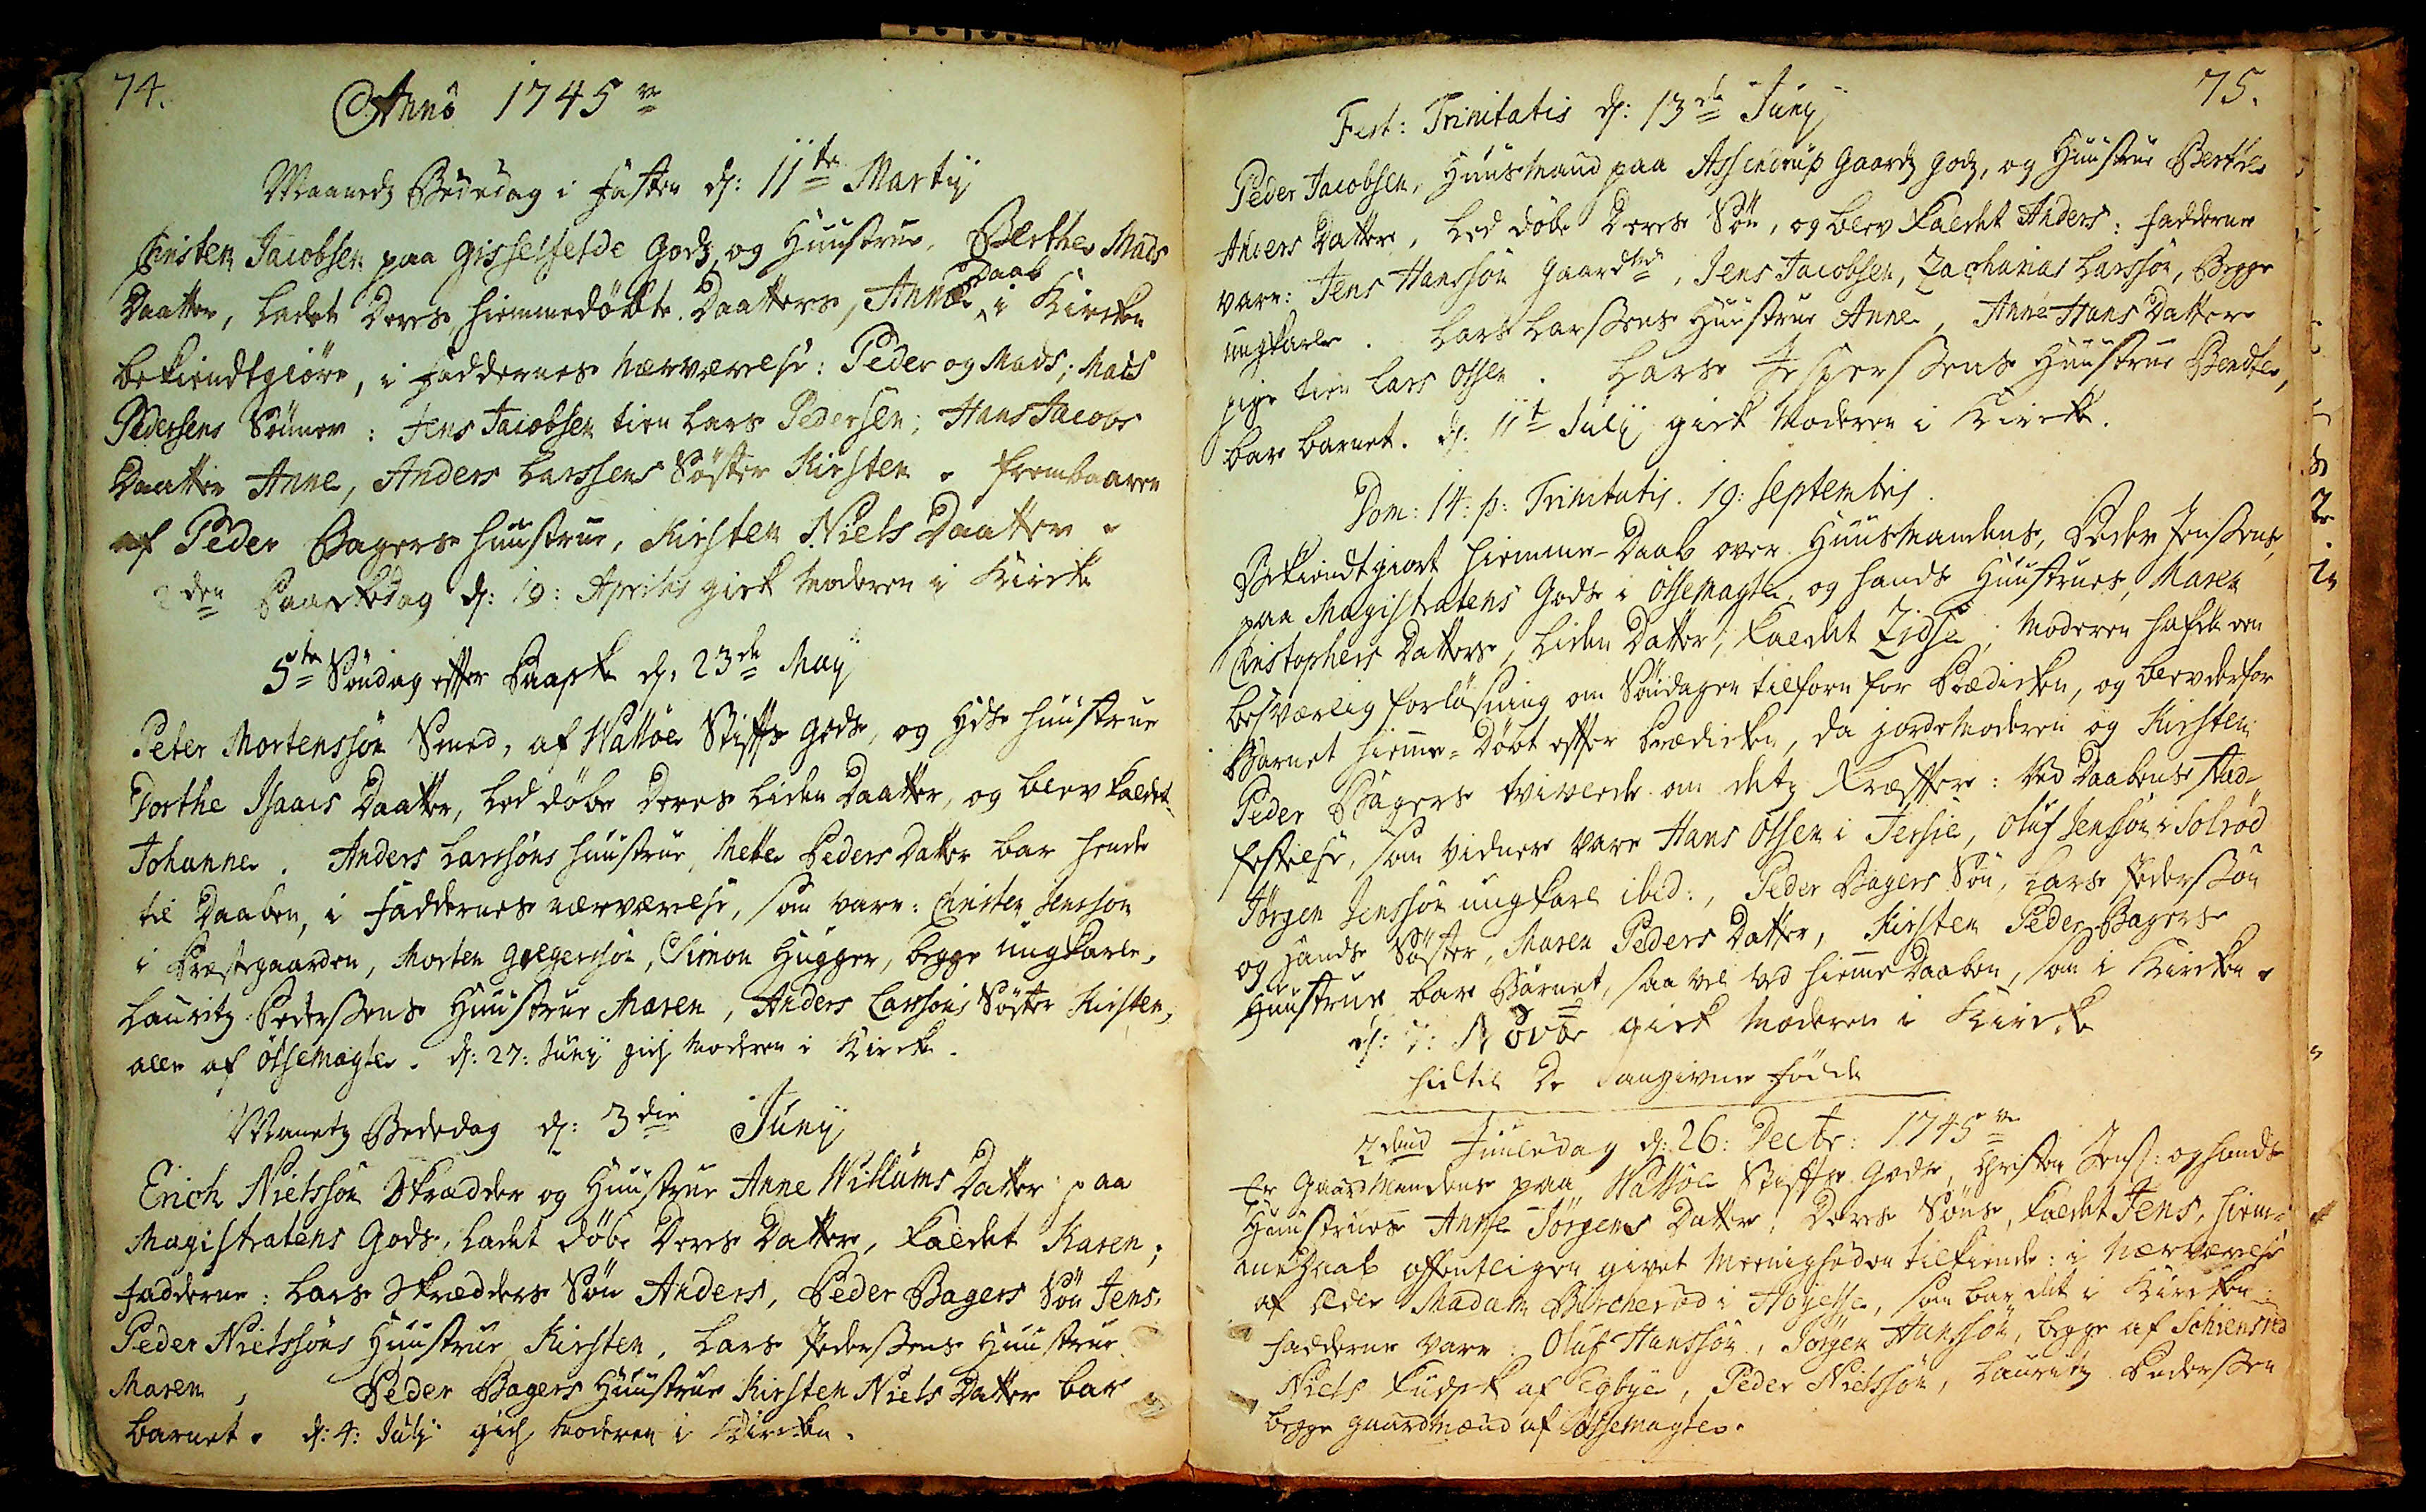74.		
Anno 1745ve
Maaneds Bededag i Fasten d: 11te Martij
Christen Jacobsen paa Gisselfelde Godz, og Huustrue, Berthe Mads
Daab
Daatter, ladet Deres hiemmedøbte Daatters, Annes i Kircken
bekiendtgiøre, i Faddernes Nærværelse: Peder og Mads; Mads
Pedersens Sønner: Jens Jacobsen tien Lars Pedersen, Hans Jacobs
Daatter Anne, Anders Larssens Søster Kirsten. Frembaaren
af Peder Bagers Huustrue, Kirsten Niels Daatter.
2den Paaskedag d: 19: Aprilis gick Moderen i Kierke.
5te Søndag eftter Paaske d: 23de Maij
Peter Mortenssøn Smed, af Walløe Stifts Gods, og hds Huustrue 
Dorthe Isaacs Daatter, lod døbe Deres liden Daatter, og blev kaldet 
Johanne. Anders Larssøns Huustrue, Mette Peders Datter bar hende
til Daaben, i Faddernes nærværelse, som vare: Christen Jensson
i Præstegaarden, Morten Gregerssøn, Simon Hugger, begge ungkarle,
Lauritz Pedersens Huustrue Maren, Anders Larssøns Søster Kirsten,
alle af Ølsemagle. D: 27: Junij gich Moderen i Kierke.
Manedz Bededag d: 3die Junij
Erich Nielssøn Skrædder og Huustrue Anne Willums Datter paa
Magistratens Gods, ladet døbe Deres Datter, kaldet Karen.
Fadderne: Lars Skrædders Søn Anders, Peder Bagers Søn Jens,
Peder Nielssøns Huustrue Kirsten, Lars Pedersens Huustrue 
Maren, Peder Bagers Huustrue Kirsten Niels Datter bar
Barnet. D. 4 Julij gick Moderen i Kierke.
75.
Fest: Trinitatis d: 13de Junij
Peder Jacobsen, Husmand paa Assendrup Gaardz Godz, og Huustrue Berthe
Anders Datter, lod døbe Deres Søn, og blev kaldet Anders: Fadderne
vare: Jens Hanssøn Gaardmd, Jens Jacobsen, Zacharias Larssøn, Begge
Ungkarle. Lars Larsens Huustrue Anne, Anne Hans Datter
pige tien Lars Olsen. Lars Jespersens Huustrue Bendte,
bar Barnet. D. 11te Julij gick Moderen i Kircke.
Dom: 14: p: Trinitatis. 19: Septembris
Bekiendtgiort Hiemme-Daab over Huusmandens, Peder Jensens,
paa Magistratens Gods i Ølsemagle, og hands Huustrues, Maren
Christophers Datters, liden Datter, kaldet Zidse. Moderen hafde en 
Besværlig Forlsning om Søndagen tilforn før Prædieken, og blev derfor
Barnet hiemme=Døbt efter Prædieken, da Jordemoderen og Kirsten
Peder Bagers tvivlede om detz Kræfter. Ved Daabens Stæd¬
fæstelse, som vidner vare Hans Olsen i Jersie, Oluf Jensson i Solrød
Jørgen Jenssøn ungkarl ibid:, Peder Bagers Søn Lars Pedersøn
Og Hands Søster, Maren Peders Datter, Kirsten Peder Bagers
Huustrue bar Barnet, saavel ved hiemme Daaben som i Kierken.
d. 7: Novbr gick Moderen i Kierke.
Hidtil De angivne fødde
2dend Juuledag d: 26 Decbr: 1745de
Er Gaardmandens paa Walløe Stifts Gods, Christen Jensen og hands
Huustrues Anne Jørgens Datter, Deres Søns, kaldet Jens, hiem¬
meDaab offentligen givet Menigheden tilkiende: i Nærværelse 
af ædle Madam Bircherod i Høyelse, som bar det i Kierken.
Fadderne vare: Oluf Hanssøn, Jørgen Hanssøn, begge af Schiensved
Niels Kudsk af Egbye, Peder Nielssøn, Lauritz Pedersen
begge Gaardmænd af Ølsemagle.